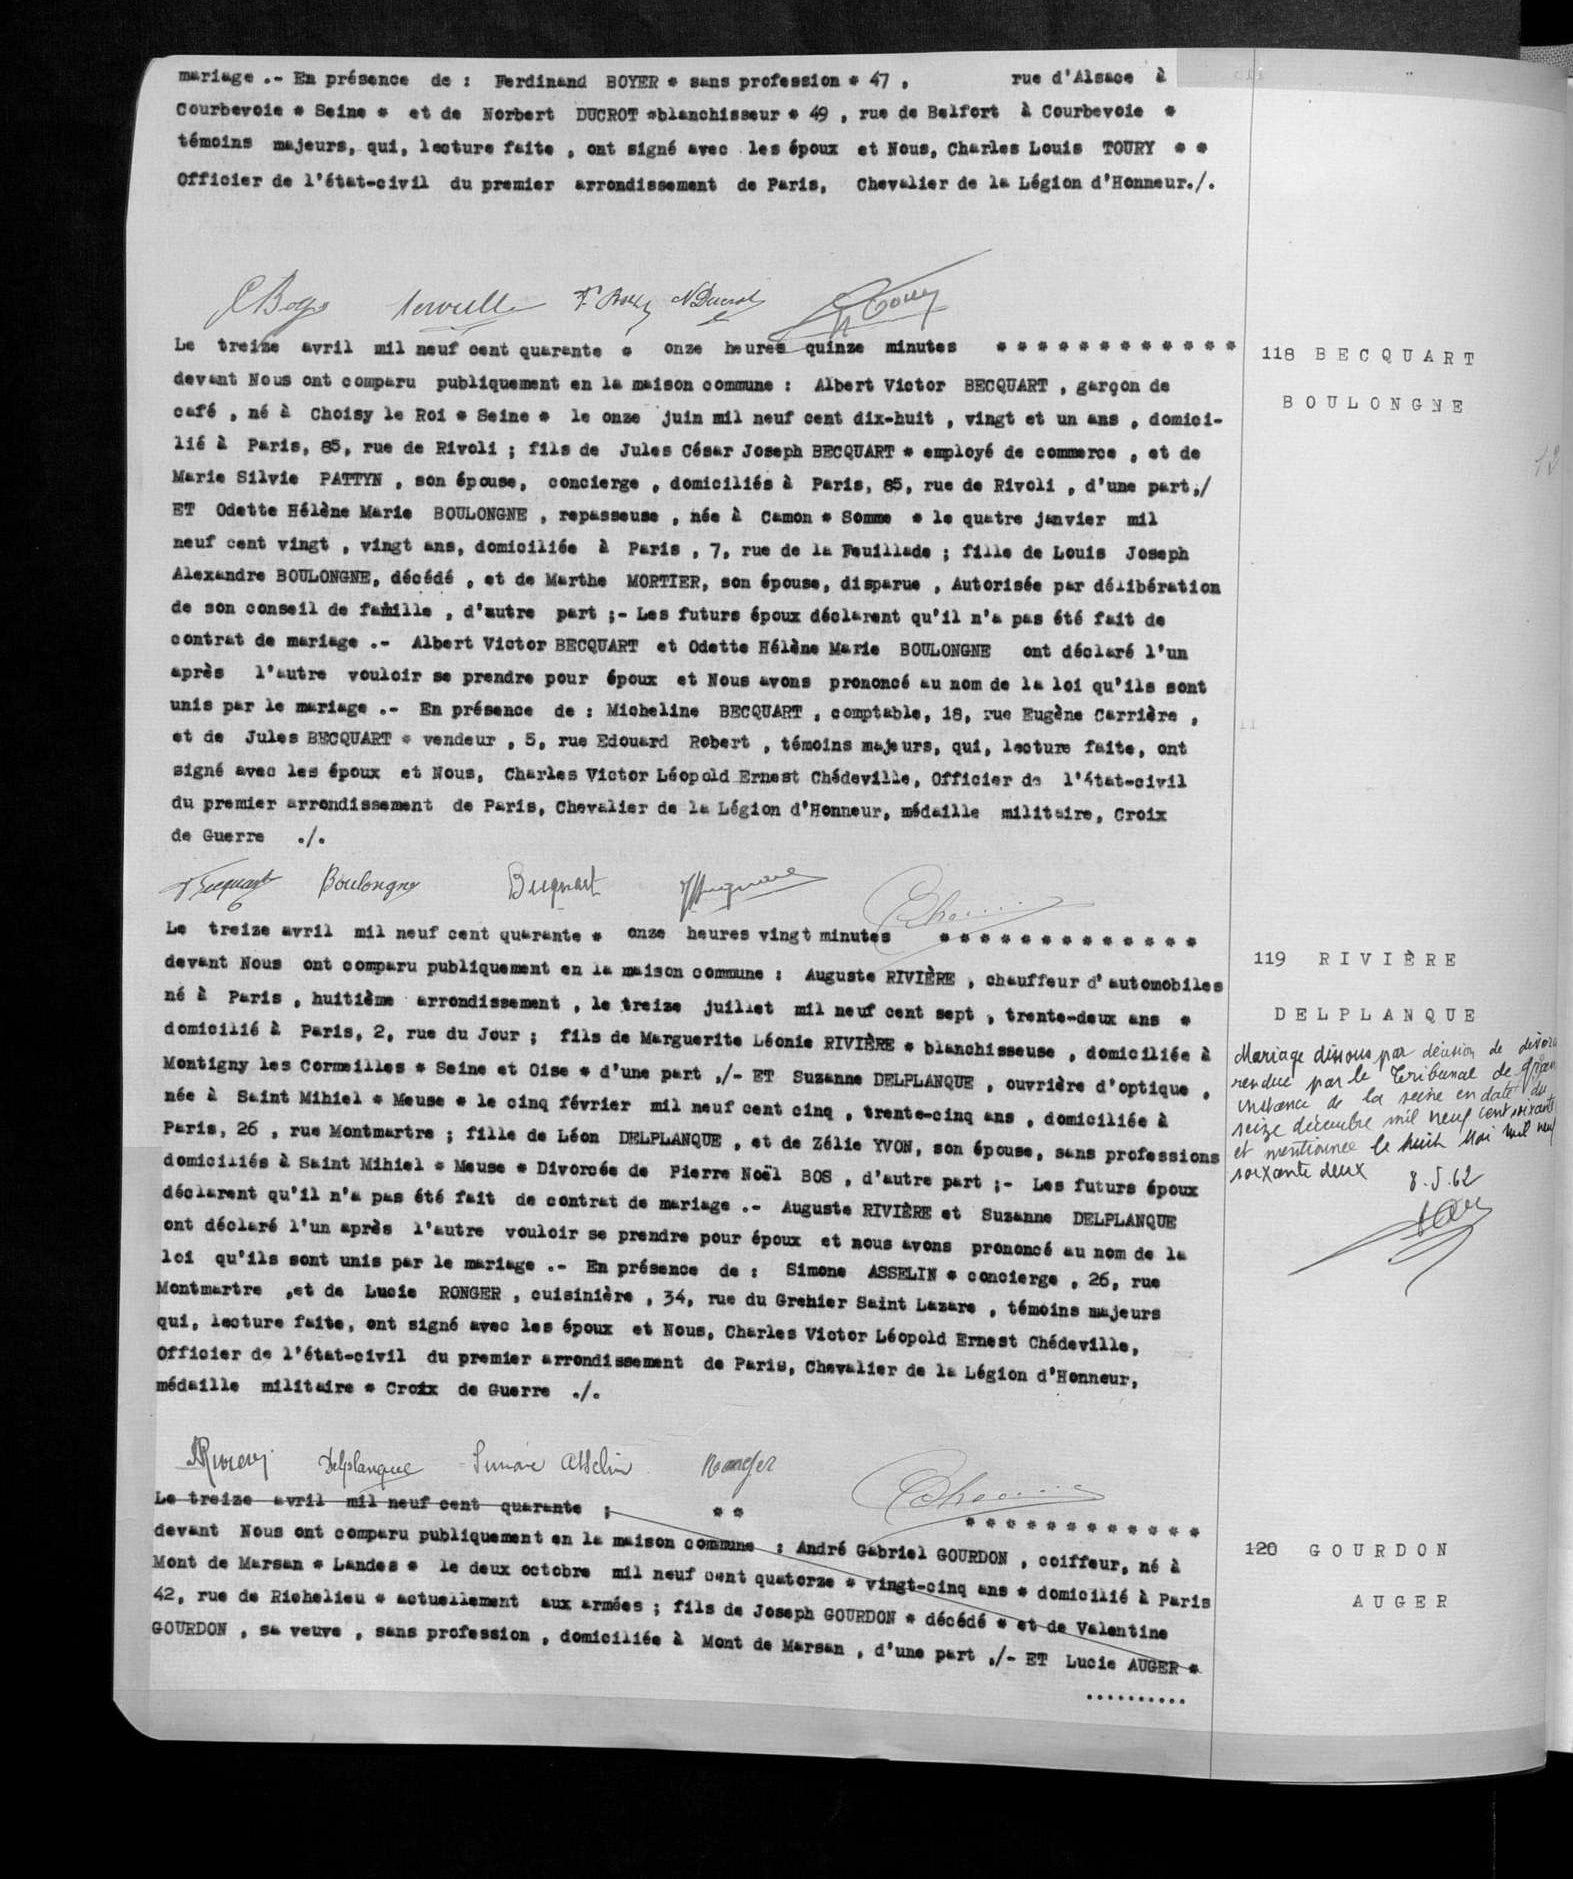mariage .-En présence de: Ferdinand BOYER * sans profession * 47, rue d'Alsace à
Courbevoie * Seine * et de Norbert DUCROT * blanchisseur * 49, rue de Belfort à Courbevoie *
témoins majeurs, qui, lecture faite, ont signé avec les époux et Nous, Charles Louis TOURY **
Officier de l'état-civil du premier arrondissement de Paris, Chevalier de la Légion d'Honneur ./.
Le treize avril mil neuf cent quarante * onze heures quinze minutes ****
devant Nous ont comparu publiquement en la maison commune: Albert Victor BECQUART, garçon de
café, né à Choisy le Roi * Seine * le onze juin mil neuf cent dix-huit, vingt et un ans, domici-
lié à Paris, 85, rue de Rivoli; fils de Jules César Joseph BECQUART * employé de commerce, et de
Marie Silvie PATTYN, son épouse, concierge, domiciliés à Paris, 85, rue de Rivoli, d'une part ,/
ET Odette Hélène Marie BOULONGNE, repasseuse, née à Camon * Somme * le quatre janvier mil
neuf cent vingt, vingt ans, domiciliée à Paris, 7, rue de la Feuillade; fille de Louis Joseph
Alexandre BOULONGNE, décédé, et de Marthe MORTIER, son épouse, disparue, Autorisée par délibération
de son conseil de famille, d'autre part ;-Les futurs époux déclarent qu'il n'a pas été fait de
contrat de mariage .-Albert Victor BECQUART et Odette Hélène Marie BOULONGNE ont déclaré l'un
après l'autre vouloir se prendre pour époux et Nous avons prononcé au nom de la loi qu'ils sont
unis par le mariage .-En présence de Micheline BECQUART, comptable, 18, rue Eugène Carrière,
et de Jules BECQUART * vendeur, 5, rue Edouard Robert, témoins majeurs, qui, lecture faite, ont
signé avec les époux et Nous, Charles Victor Léopold Ernest Chédeville, Officier de l'état-civil
du premier arrondissement de Paris, Chevalier de la Légion d'Honneur, médaille militaire, Croix
de Guerre ./.
Le treize avril mil neuf cent quarante * onze heures vingt minutes ****
devant Nous ont comparu publiquement en la maison commune: Auguste RIVIERE, chauffeur d'automobiles
né à Paris, huitième arrondissement, le treize juillet mil neuf cent sept, trente-deux ans *
domicilié à Paris, 2, rue du Jour; fils de Marguerite Léonie RIVIERE * blanchisseuse, domicilié à
Montigny les Cormeille * Seine et Oise * d'une part ,/-ET Suzanne DELPLANQUE, ouvrière d'optique,
née à Saint Mihiel * Meuse * le cinq février mil neuf cent cinq, trente-cinq ans, domicilié à
Paris, 26, rue Montmartre; fille de Léon DELPLANQUE, et de Zélie YVON, son épouse, sans professions
domiciliés à Saint Mihiel * Meuse * Divorcée de Pierre Noël BOS, d'autre part;-Les futurs époux
déclarent qu'il n'a pas été fait de contrat de mariage .-Auguste RIVIERE et Suzanne DELPLANQUE
ont déclaré l'un après l'autre vouloir se prendre pour époux et nous avons prononcé au nom de la
loi qu'ils sont unis par le mariage .-En présence de: Simone ASSELIN * concierge, 26, rue
Montmartre, et de Lucie RONGER, cuisinière, 34, rue du Grehier Saint Lazare, témoins majeurs
qui, lecture faite, ont signé avec les époux et Nous, Charles Victor Léopold Ernest Chédeville,
Officier de l'état-civil du premier arrondissement de Paris, Chevalier de la Légion d'Honneur,
médaille militaire * Croix de Guerre ./.
Le treize avril mil neuf cent quarante; ****
devant Nous ont comparu publiquement en la maison commune: André Gabriel GOURDON, coiffeur, né à
Mont de Marsan * Landes * le deux octobre mil neuf cent quatorze * vingt-cinq ans * domicilié à Paris
42, rue de Richelieu * actuellement aux armées; fils de Joseph GOURDON * décédé * et de Valentine
GOURDON, sa veuve, sans profession, domiciliée à Mont de Marsan, d'une part ,/-ET Lucie AUGER *
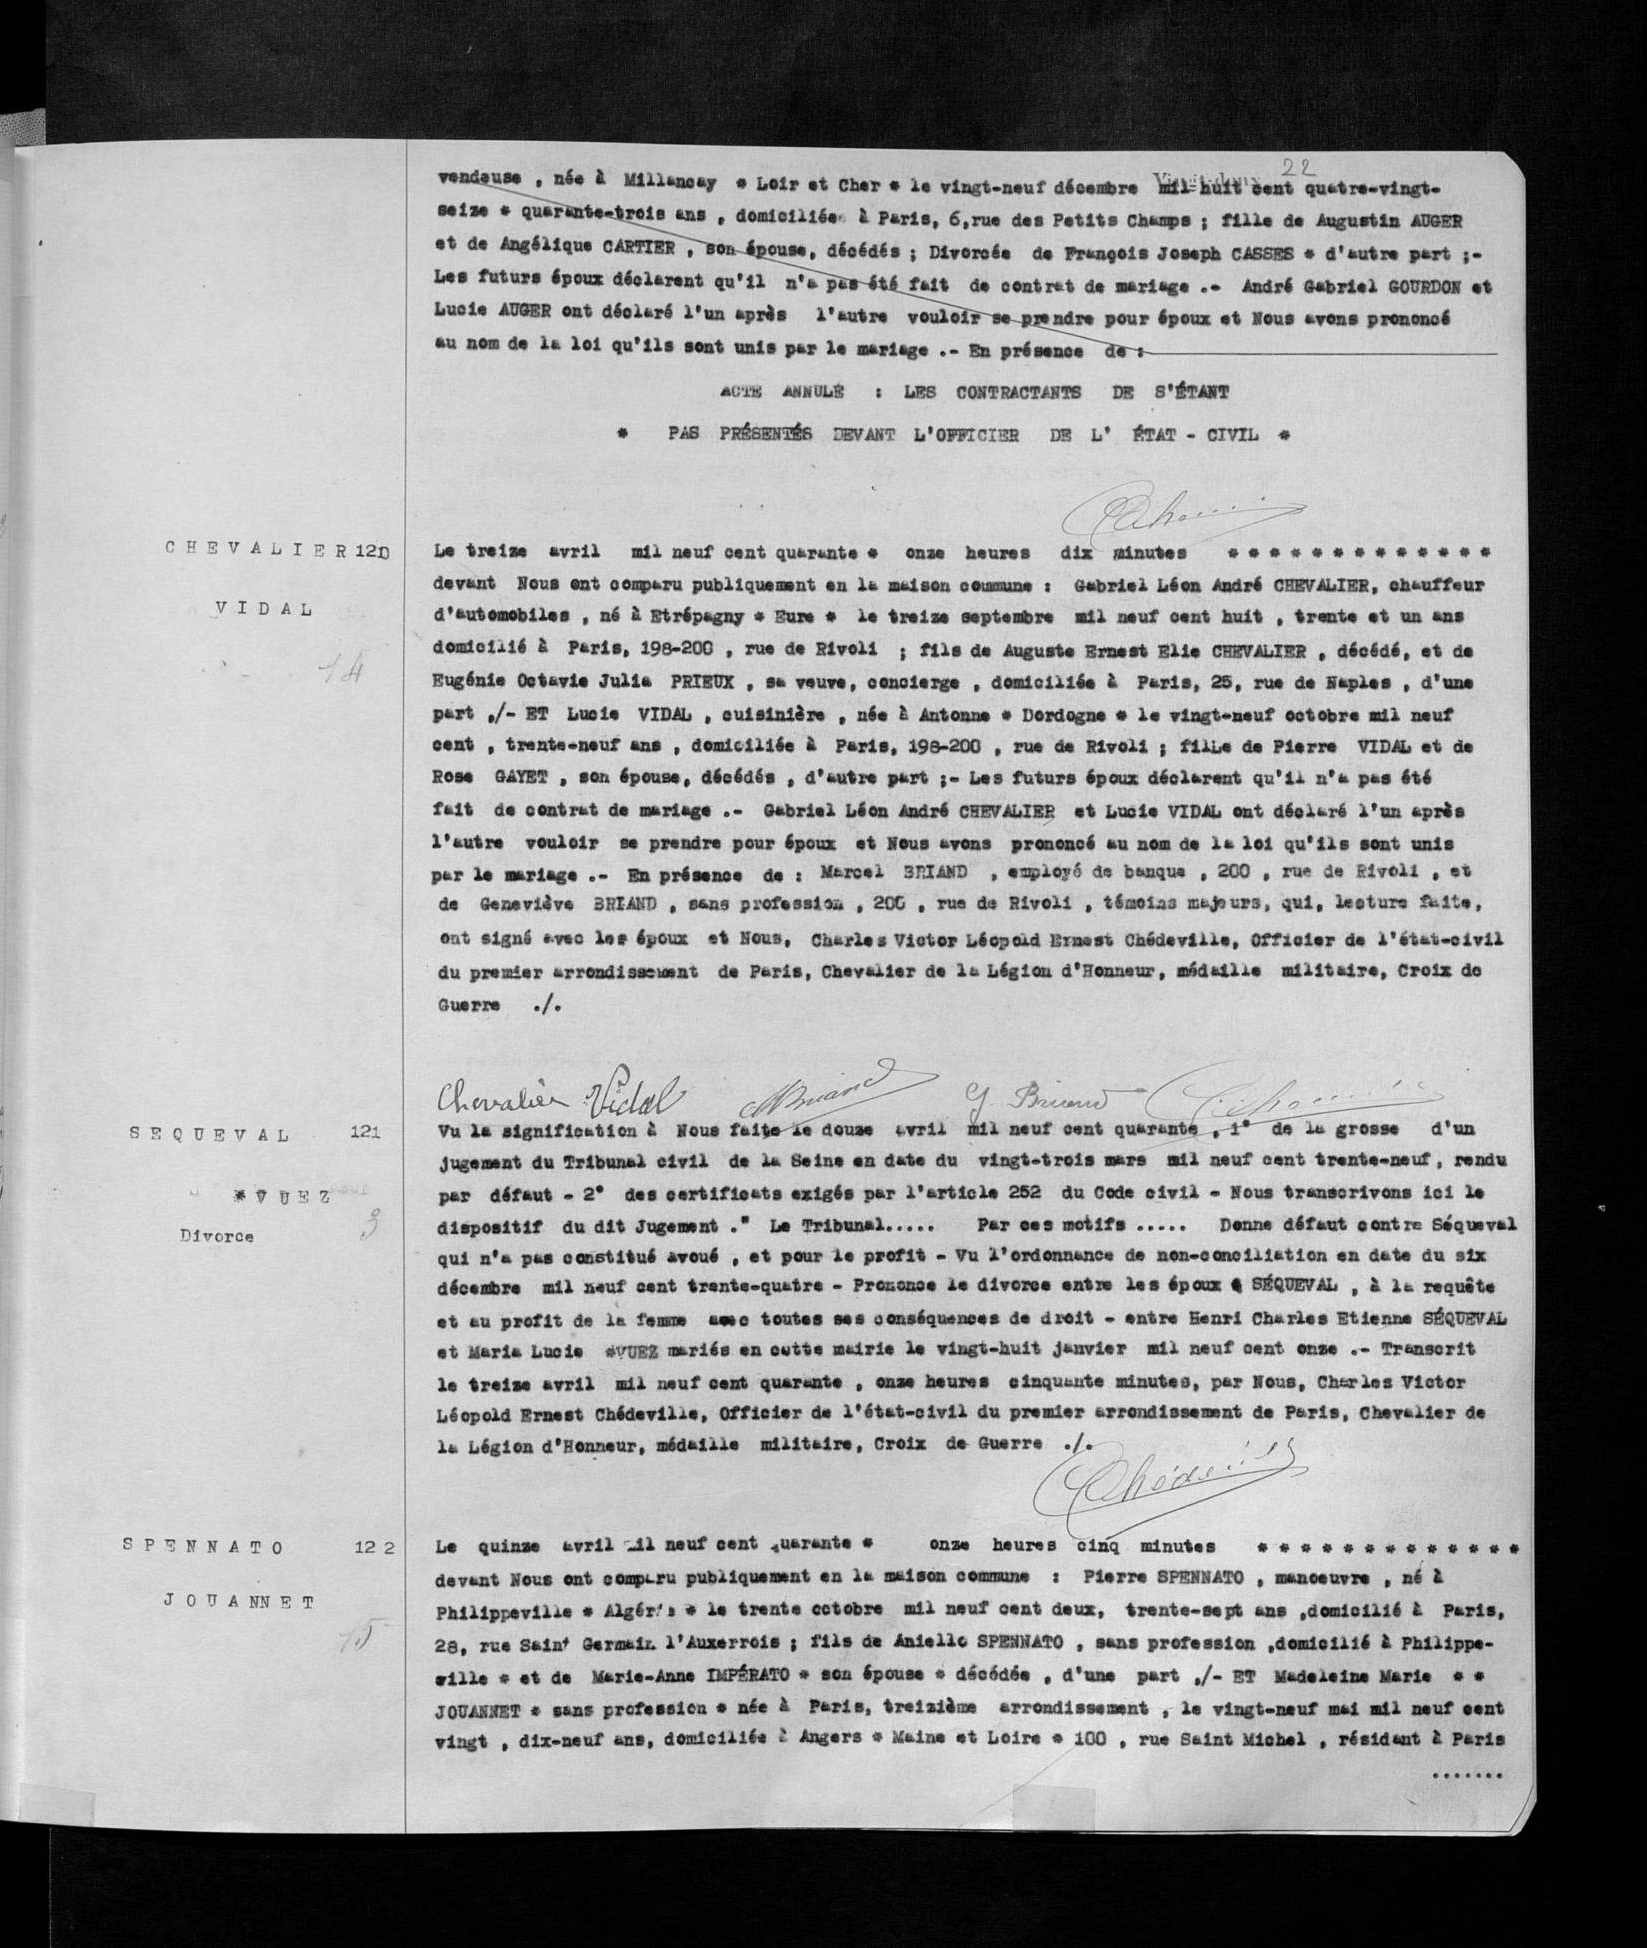vendeuse, née à Millancay * Loir et Cher * le vingt-neuf décembre mil-huit cent quatre-vingt-
seize * quarante-trois ans, domiciliée à Paris, 6, rue des Petits Champs; fille de Augustin AUGER
et de Angélique CARTIER, son épouse, décédés; Divorcée de François Joseph CASSES * d'autre part;
Les futurs époux déclarent q'iil n'a pas été fait de contrat de mariage .-André Gabriel GOURDON et
Lucie AUGER ont déclaré l'un après l'autre vouloir se prendre pour époux et Nous avons prononcé
au nom de la loi qu'ils sont unis par le mariage .-En présence de:
ACTE ANNULE: LES CONTRACTANTS DE S'ETANT
* PAS PRESENTES DEVANT L'OFFICIER DE L'ETAT-CIVIL *
Le treize avril mil neuf cent quarante * onze heures dix minutes ****
devant Nous ont comparu publiquement en la maison commune: Gabriel Léon André CHEVALIER, chauffeur
d'automobiles, né à Etrépagny * Eure * le treize septembre mil neuf cent huit, trente et un ans
domicilié à Paris, 198-200, rue de Rivoli; fils de Auguste Ernest Elie CHEVALIER, décédé, et de
Eugénie Octavie Julia PRIEUX, sa veuve, concierge, domiciliée à Paris, 25, rue de Naples, d'une
part ,/-ET Lucie VIDAL, cuisinière, née à Antonne * Dordogne * le vingt-neuf octobre mil neuf
cent, trente-neuf ans, domiciliée à Paris, 198-200, rue de Rivoli, fille de Pierre VIDAL et de
Rose GAYET, son épouse, décédés, d'autre part ;-Les futurs époux déclarent qu'il n'a pas été
fait de contrat de mariage .-Gabriel Léon André CHEVALIER et Lucie VIDAL ont déclaré l'un après
l'autre vouloir se prendre pour époux et Nous avons prononcé au nom de la loi qu'ils sont unis
par le mariage .-En présence de: Marcel BRIAND, employé de banque, 200, rue de Rivoli, et
de Geneviève BRIAND, sans profession, 200, rue de Rivoli, témoins majeurs, qui, lecture faite,
ont signé avec les époux et Nous, Charles Victor Léopold Ernest Chédeville, Officier de l'état-civil
du premier arrondissement de Paris, Chevalier de la Légion d'Honneur, médaille militaire, Croix de
Guerre ./.
Vu la signification à Nous faite le douze avril mil neuf cent quarante, 1° de la grosse d'un
jugement du Tribunal civil de la Seine en date du vingt-trois mars mil neuf cent trente-neuf, rendu
par défaut-2° des certificats exigés par l'article 252 du Code civil-Nous transcrivons ici le
dispositif du dit Jugement. "Le Tribunal..... Par ces motifs..... Donne défaut contre Séqueval
qui n'a pas constitué avoué, et pour le profit-Vu l'ordonnance de non-conciliation en date du six
décembre mil neuf cent trente-quatre-Prononce le divorce entre les époux SEQUEVAL, à la requête
et au profit de la femme avec toutes ses conséquences de droit-entre Henri Charles Etienne SEQUEVAL
et Maria Lucie *VUEZ mariés en cette mairie le vingt-huit janvier mil neuf cent onze .-Transcrit
le treize avril mil neuf cent quarante, onze heures cinquante minutes, par Nous, Charles Victor
Léopold Ernest Chédeville, Officier de l'état-civil du premier arrondissement de Paris, Chevalier de
la Légion d'Honneur, médaille militaire, Croix de Guerre ./.
Le quinze avril mil neuf cent quarante * onze heures cinq minutes ****
devant Nous ont comparu publiquement en la maison commune: Pierre SPENNATO, manoeuvre, né à
Philippeville * Algérie * le trente octobre mil neuf cent deux, trente-sept ans, domicilié à Paris,
28, rue Saint Germain l'Auxerrois; fils de Aniello SPENNATO, sans profession, domicilié à Phillippe-
ville * et de Marie-Anne IMPERATO * son épouse * décédée, d'une part ,/-ET Madeleine Marie **
JOUANNET * sans profession * née à Paris, treizième arrondissement, le vingt-neuf mai mil neuf cent
vingt, dix-neuf ans, domiciliée à Angers * Maine et Loire * 100, rue Saint Michel, résidant à Paris